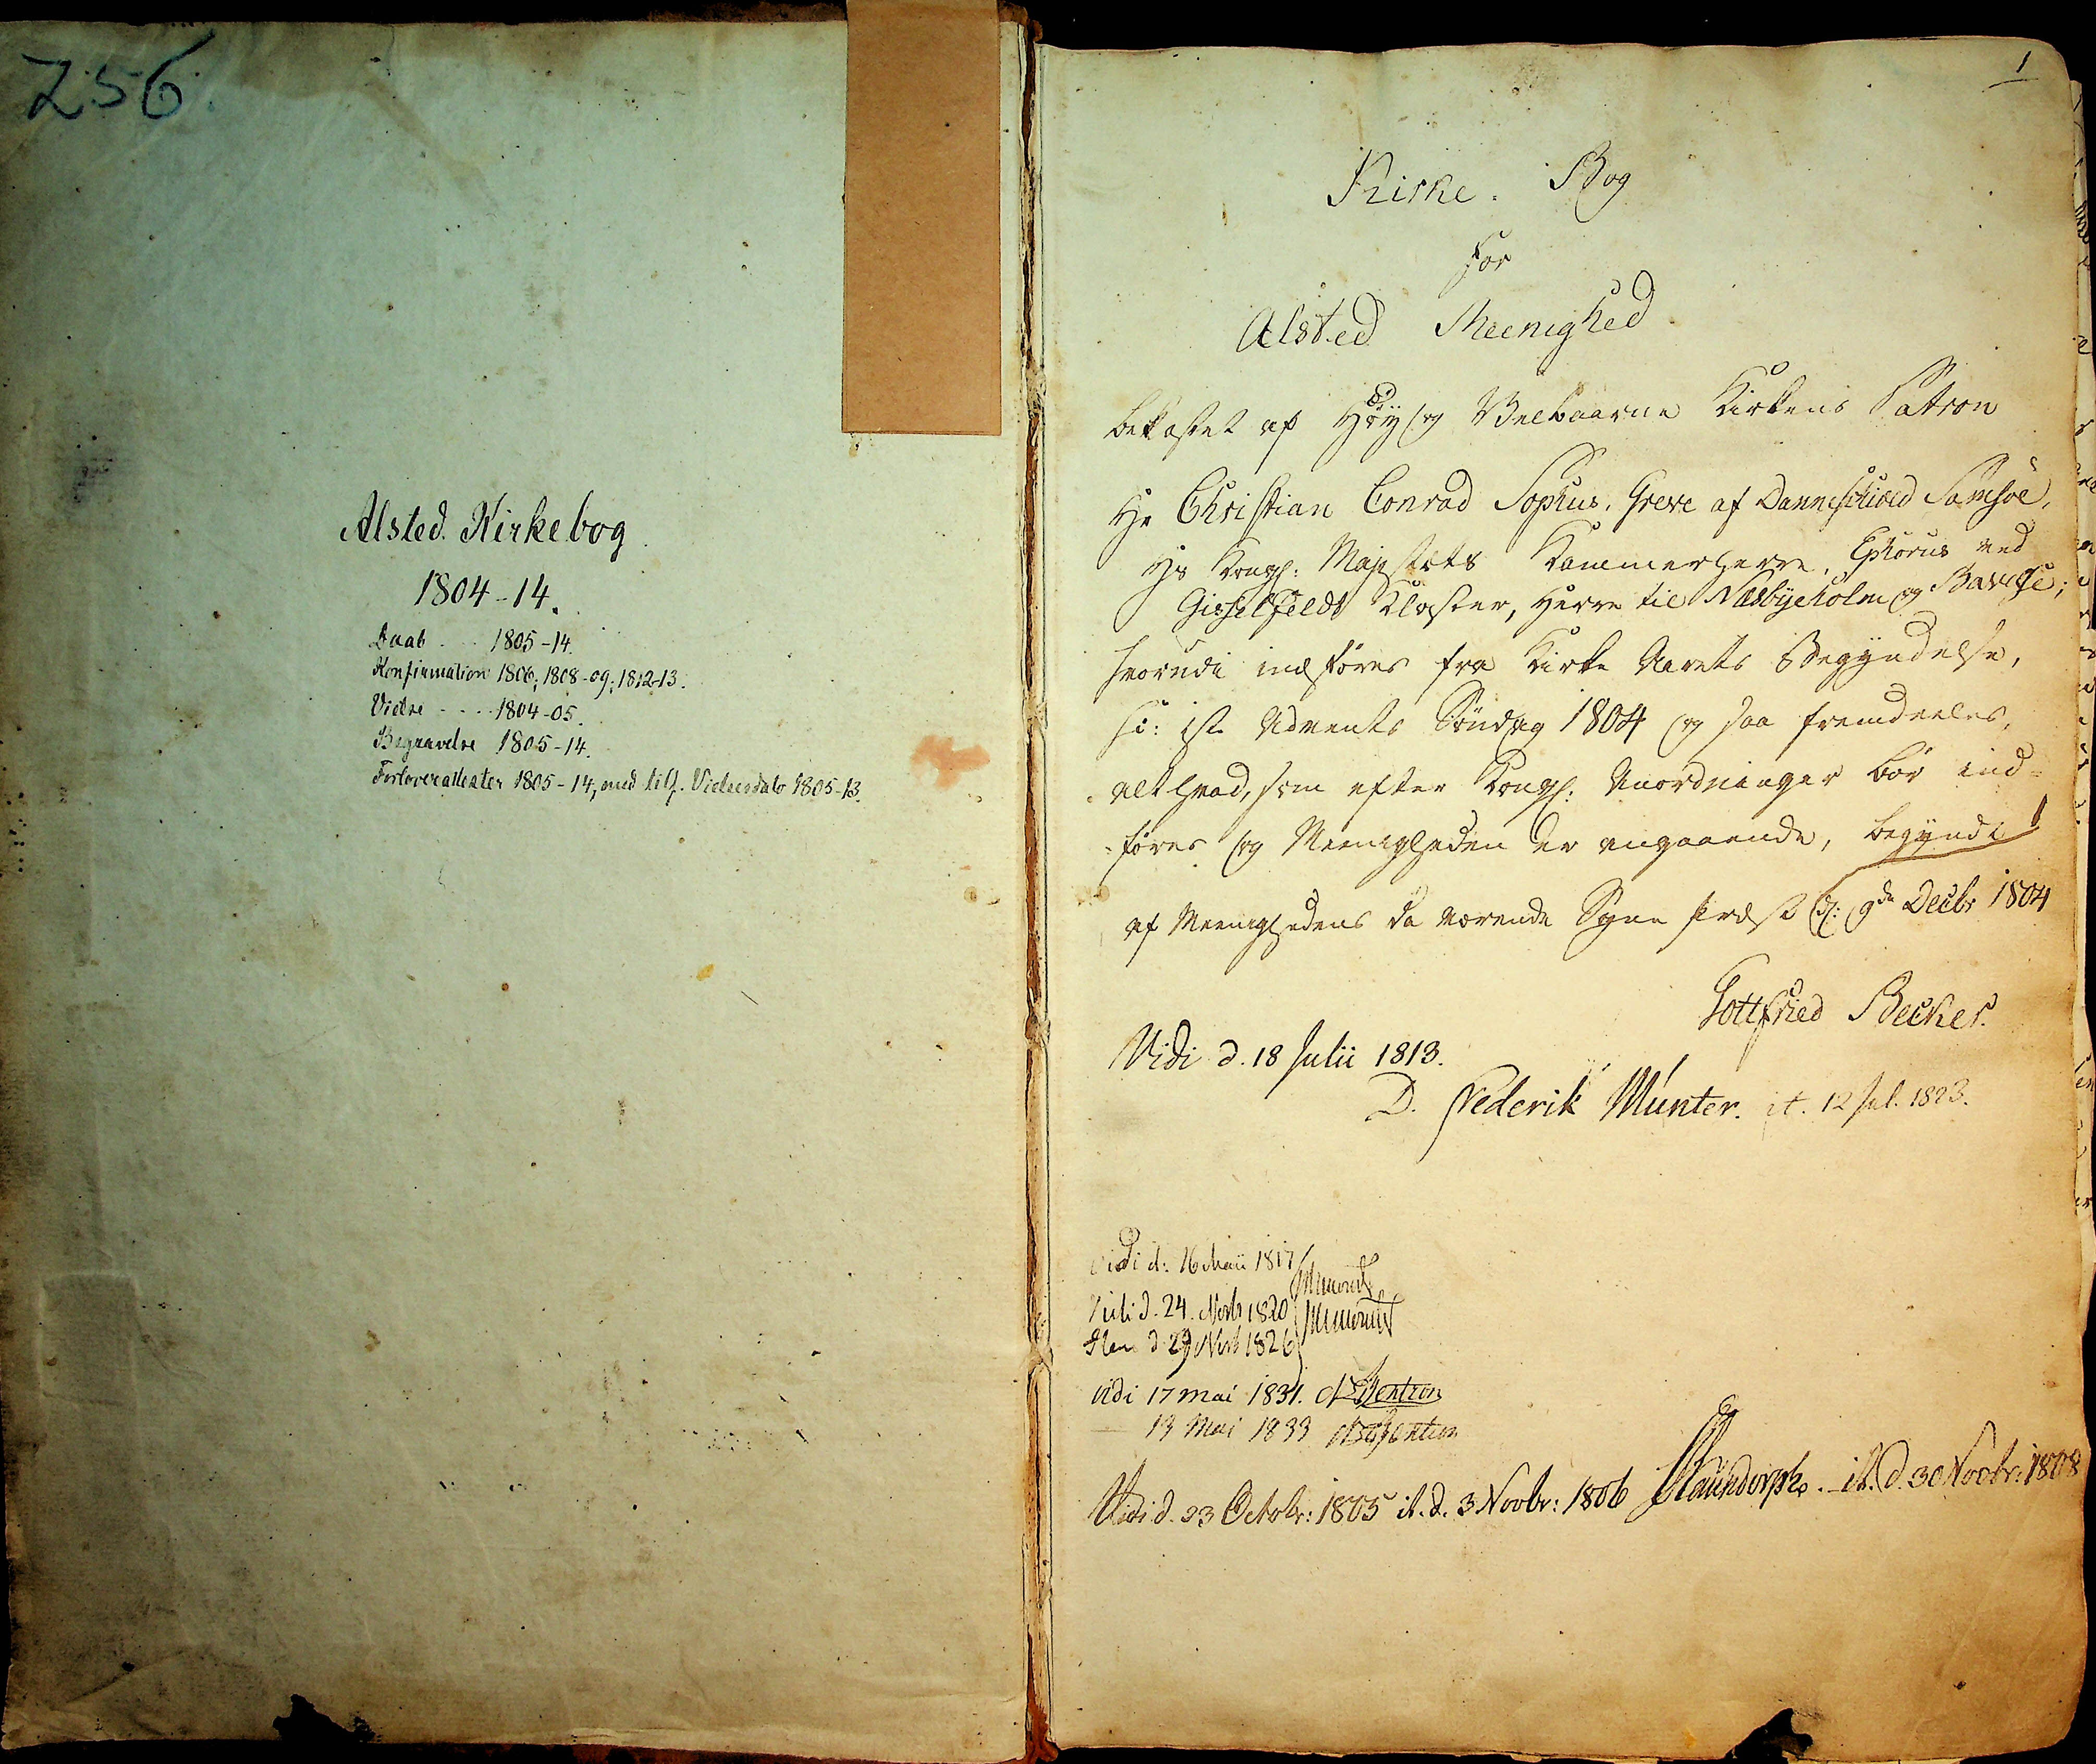1
Kirke Bog
for
Alsted Meenighed.
Bekostet af Høy og Velbaarne Kirkens Patron
Hr Christian Conrad Sophus Greve af Danneschiold Samsøe
Hr Kongl: Majatæts Kammerherre, Ephorus ved
Gisselfeldt Kloster, Herre til Næsbyeholm og Bavelse;
hvorudi indføres fra Kirke Aarets Begyndelse,
sc: 1ste Advents Søndag 1804 og saa fremdeeles
ret hvad, som efter Kongl: Anordninger bor ind¬
føres og Meenigheden er angaaende, begyndt
d: 9de Decbr 1804
af Meenighedens da værende Sogne præst 
Gottfried Becker
Vidi d. 18 Julii 1813
D. Frederik Munter. it. 22 Jul. 1823
Vidi d: 16 Maii 1812
Vidi d. 24. Novb 1820
Item d 29 Novb 1826
##
##
vidi 17 Mai 1831. N Bentzon
13 Mai 1823 N Bentzon
Vidi d. 23. Octobr: 1805 it. 3 Novbr: 1806. ##aundorph. it. d. 30 Novbr: 1808
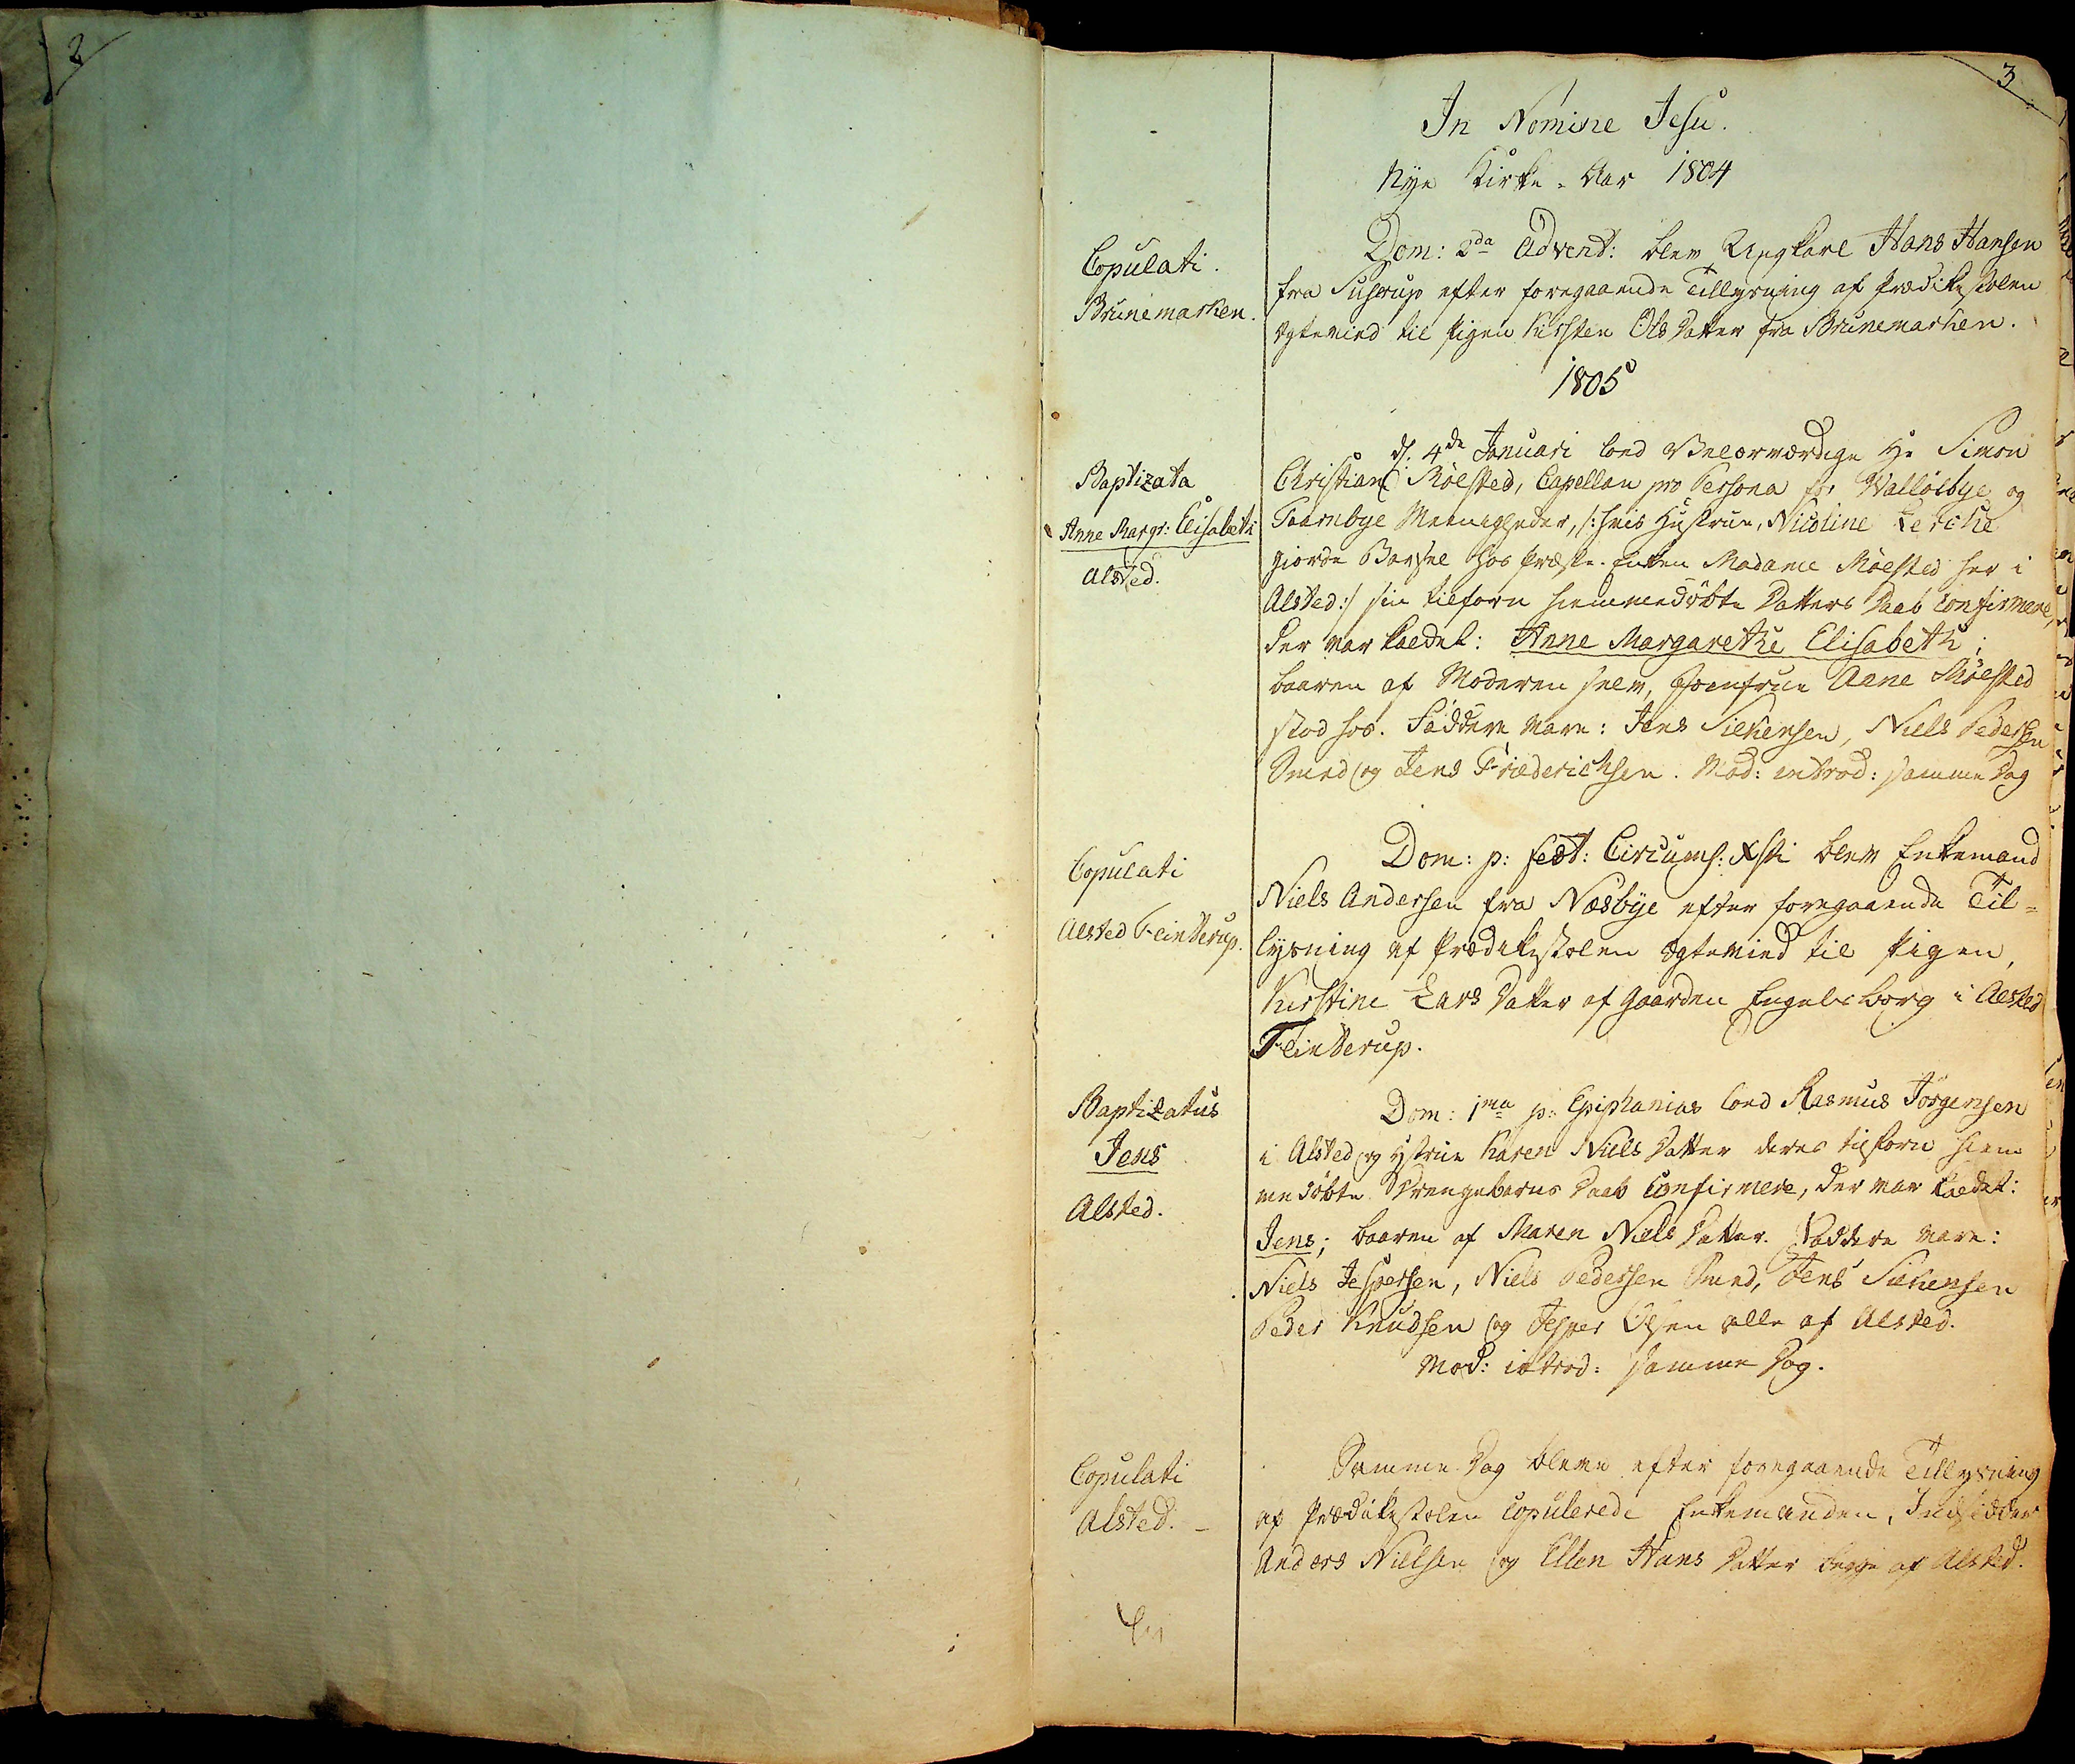Copulati
Brunemarken
Baptizata
Anne Margr: Elisabeth
Alsted.
Copulato
UAlsted Flinterup.
Baptizatus
Jens
Alsted.
Copulati
Alsted.
##
3
In Nomine Jesu.
Nye Kirke - Aar 1804
Dom: 2da Advent: blev Ungkarl Hans Hansen
fra Suserup efter foregaaende Tillysning af Prædikestolen
Ægtevied til Pigen Kirsten Ols Datter fra Brunemarken.
1805
d: 4de Januari loed Velærværdige Hr Simon
Christian Mølsted, Capellan pro Persona for Walløebye og
Taarnbye Meenigheder, /: hvis Hustrue, Nicoline Lerche
giorde Barsel hos Præste Enken Madame Mølsted her i
Alsted :/ sin tilforn hiemmedøbte Datters Daab confirmere
der var kaldet Anne Margarethe Elisabeth;
baaren af Moderen selv, Jomfrue Anne Mølsted
stod hos. Faddere vare: Jens Silkensen, Niels Pedersen
Smed og Jens Fræderichsen, Mod: introd: samme Dag
Dom: p: Fest: Circums: Xsti blev Enkemand
Niels Andersen fra Næsbye efter foregaaende Til¬
Lysning af Prædikestolen ægtevied til Pigen,
Kirstine Lars Datter af Gaarden Engels borg i Alsted
Flinterup.
Dom: 1ma p. Epiphanias loed Rasmus Jørgensen
i Alsted og Hustrue Karen Niels Datter deres tilforn hiem¬
medøbte Drengebarns Daab Confirmere, der var kaldet:
Jens, baaren af Maren Niels Datter. Faddere vare:
Niels Jespersen, Niels Pedersen Smed, Jens Silkensen
Peder Knudsen og Jesper Olsen alle af Alsted.
Mod: introd: samme Dag.
Samme Dag bleve efter foregaaende Tillysning
af Prædikestolen copulerede Enkemanden Indsidder
Anders Nielsen og Ellen Hans Datter begge af Alsted.